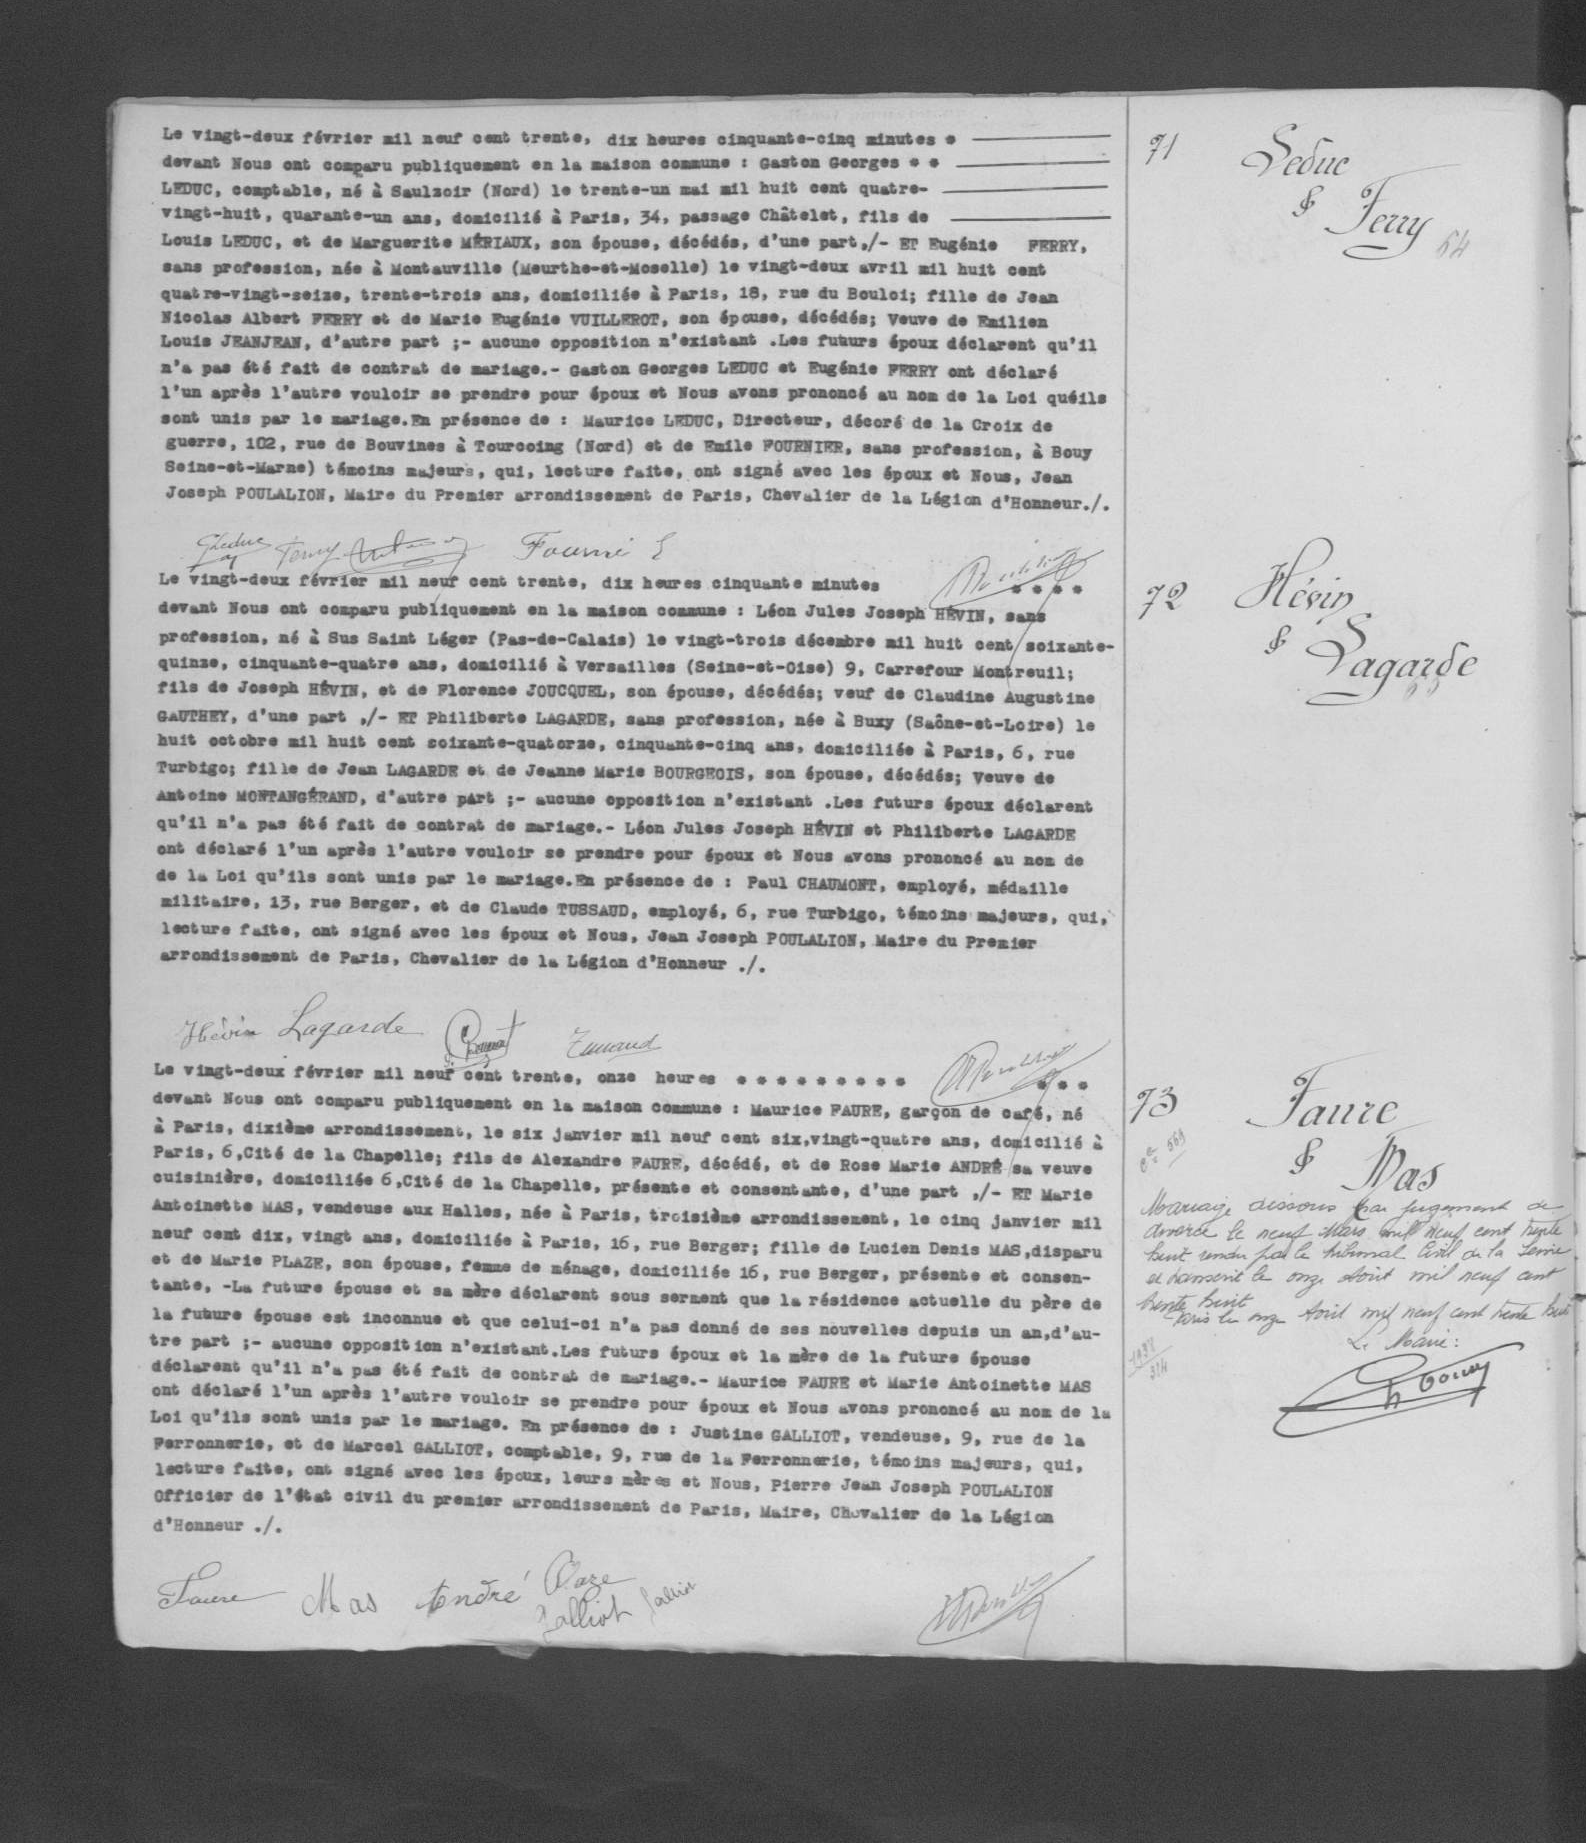Le vingt-deux février mil neuf cent trente, dix heures cinquante-cinq minutes * -
devant Nous ont comparu publiquement en la maison commune: Gaston Georges * * -
LEDUC, comptable, né à Saulzoir (Nord) le trente-un mai mil huit cent quatre-
vingt-huit, quarante-un ans, domicilié à Paris, 34, passage Châtelet, fils de -
Louis LEDUC, et de Marguerite MERIAUX, son épouse, décédés, d'une part ,-/ ET Eugénie FERRY,
sans profession, née à Montauville (Meurthe-et-Moselle) le vingt-deux avril mil huit cent
quatre-vingt-seize, trente-trois ans, domiciliée à Paris, 18, rue du Bouloi; fille de Jean
Nicolas Albert FERRY et de Marie Eugénie VUILLEROT, son épouse, décédés; veuve de Emilien
Louis JEANJEAN, d'autre part ;-aucune opposition n'existant. Les futurs époux déclarent qu'il
n'a pas été fait de contrat de mariage .-Gaston Georges LEDUC et Eugénie FERRY ont déclaré
l'un après l'autre vouloir se prendre pour époux et Nous avons prononcé au nom de la Loi quéils
sont unis par le mariage. En présence de: Maurice LEDUC, Directeur, décoré de la Croix de
guerre, 102, rue de Bouvines à Tourcoing (Nord) et de Emile FOURNIER, sans profession, à Bouy
Seine-et-Marne) témoins majeurs, qui, lecture faite, ont signé avec les époux et Nous, Jean
Joseph POULALION, Maire du Premier arrondissement de Paris, Chevalier de la Légion d'Honneur ./.
Le vingt-deux février mil neuf cent trente, dix heures cinquante minutes ****
devant Nous ont comparu publiquement en la maison commune: Léon Jules Joseph HEVIN, sans
profession, né à Sus Saint Léger (Pas-de-Calais) le vingt-trois décembre mil huit cent soixante-
quinze, cinquante-quatre ans, domicilié à Versailles (Seine-et-oise) 9, Carrefour Montreuil;
fils de Joseph HEVIN, et de Florence JOUCQUEL, son épouse, décédés; veuf de Claudine Augustine
GAUTHEY, d'une part ,/-ET Philiberte LAGARDE, sans profession, née à Buxy (Saône-et-Loire) le
huit octobre mil huit cent soixante-quatorze, cinquante-cinq ans, domiciliée à Paris, 6, rue
Turbigo; fille de Jean LAGARDE et de Jeanne Marie BOURGEOIS, son épouse, décédés; veuve de
Antoine MONTANGERAND, d'autre part ;-aucune opposition n'existant. Les futurs époux déclarent
qu'il n'a pas été fait de contrat de mariage .-Léon Jules Joseph HEVIN et Philiberte LAGARDE
ont déclaré l'un après l'autre vouloir se prendre pour époux et Nous avons prononcé au nom de
de la Loi qu'ils sont unis par le mariage. En présence de: Paul CHAUMONT, employé, médaille
militaire, 13, rue Berger, et de Claude TUSSAUD, employé, 6, rue Turbigo, témoins majeurs, qui,
lecture faite, ont signé avec les époux et Nous, Jean Joseph POULALION, Maire du Premier
arrondissement de Paris, Chevalier de la Légion d'Honneur ./.
Le vingt-deux février mil neuf cent trente, onze heures ****
devant Nous ont comparu publiquement en la maison commune: Maurice FAURE, garçon de café, né
à Paris, dixième arrondissement, le dix janvier mil neuf cent six, vingt-quatre ans, domicilié à
Paris, 6, Cité de la Chapelle; fils de Alexandre FAURE, décédé, et de Rose Marie ANDRE sa veuve
cuisinière, domiciliée 6, Cité de la Chapelle, présente et consentante, d'une part ,/-ET marie
Antoinette MAS, vendeuse aux Halles, née à Paris, troisième arrondisement, le cinq janvier mil
neuf cent dix, vingt ans, domiciliée à Paris, 16, rue Berger; fille de Lucien Denis MAS, disparu
et de Marie PLAZE, son épouse, femme de ménage, domiciliée 16, rue Berger, présente et consen-
tante,-La future épouse et sa mère déclarent sous serment que la résidence actuelle du père de
la future épouse est inconnue et que celui-ci n'a pas donné de ses nouvelles depuis un an, d'au-
tre part ;-aucune opposition n'existant. Les futurs époux et la mère de la future épouse
déclarent qu'il n'a pas été fait de contrat de mariage.-Maurice FAURE et Marie Antoinette MAS
ont déclaré l'un après l'autre vouloir se prendre pour époux et Nous avons prononcé au nom de la
Loi qu'ils sont unis par le mariage. En présence de: Justine GALLIOT, vendeuse, 9, rue de la
Ferronnerie, et de Marcel GALLIOT, comptable, 9, rue de la Ferronnerie, témoins majeurs, qui,
lecture faite, ont signé avec les époux, leurs mères et Nous, Pierre jean Joseph POULALION
Officier de l'état civil du premier arrondissement de Paris, Maire, Chevalier de la Légion
d'Honneur ./.
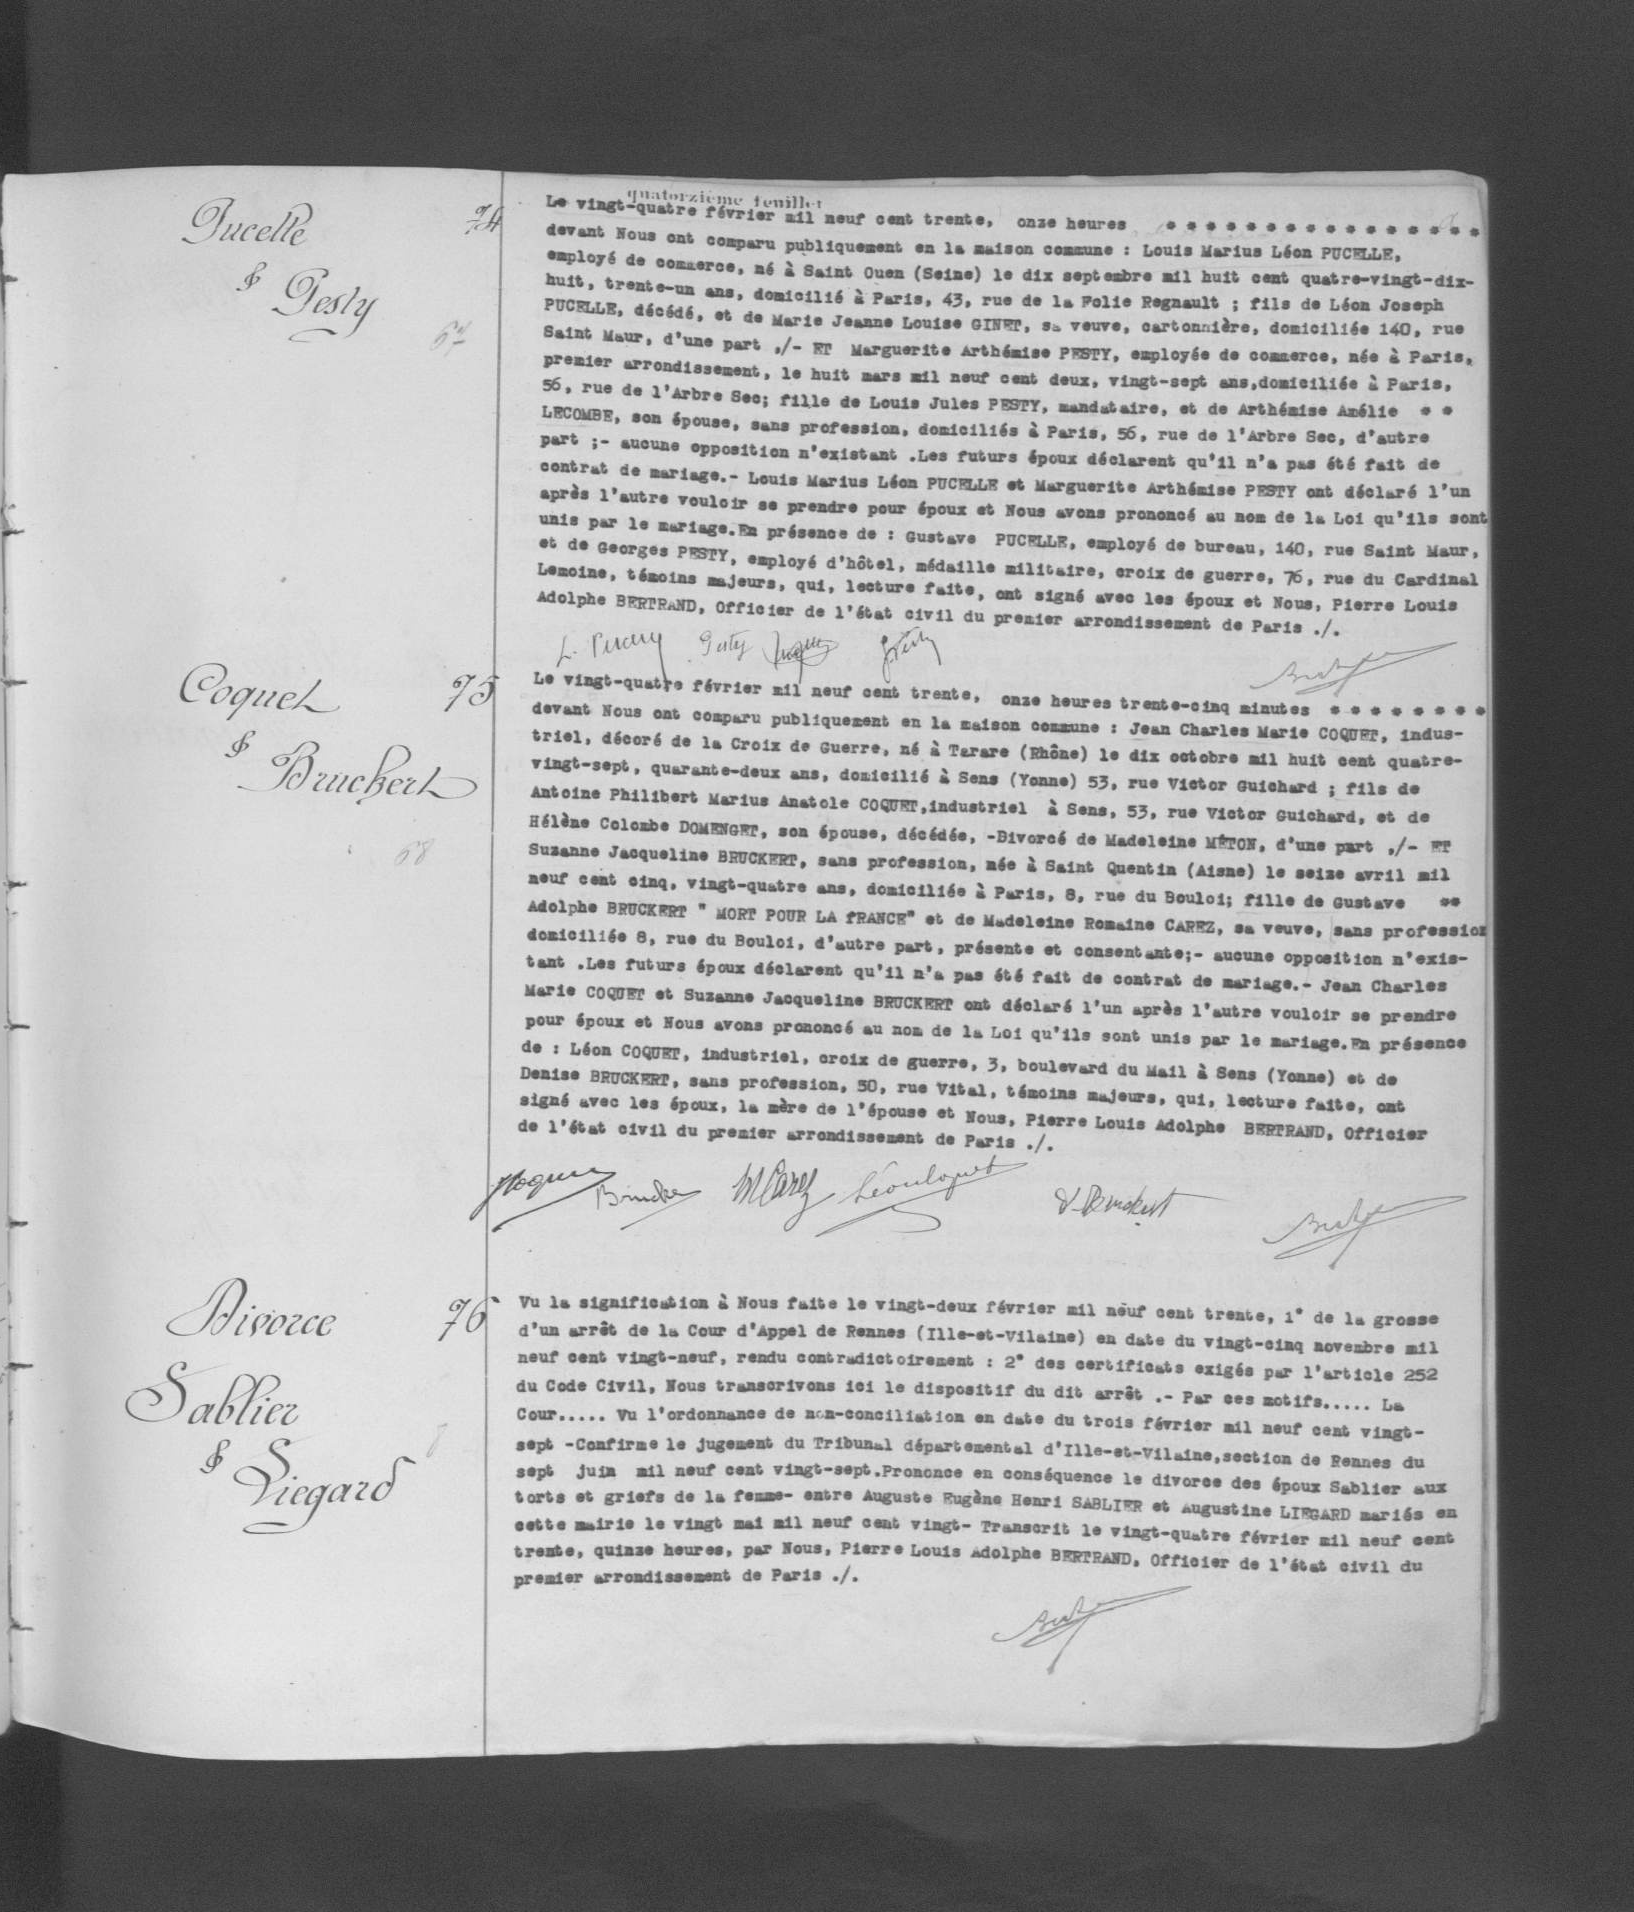Le vingt-quatre février mil neuf cent trente, onze heures ****
devant Nous ont comparu publiquement en la maison commune: Louis Marius Léon PUCELLE,
employé de commerce, né à Saint Ouen (Seine) le dix septembre mil huit cent quatre-vingt-dix-
huit, trente-un ans, domicilié à Paris, 43, rue de la Folie Regnault; fils de Léon Josep
PUCELLE, décédé, et de Marie Jeanne Louise GINET, sa veuve, cartonnière, domiciliée 140, rue
Saint Maur, d'une part ,/-ET Marguerite Arthémise PESTY, employée de commerce, née à Paris,
premier arrondissement, le huit mars mil neuf cent deux, vingt-sept ans, domiciliée à Paris,
56, rue de l'Arbre Sec; fille de Louis Jules PESTY, mandataire, et de l'Arthémise Amélie * *
LECOMBE, son épouse, sans profession, domiciliés à Paris, 56, rue de l'Arbre Sec, d'autre
part ;-aucune opposition n'existant. Les futurs époux déclarent qu'il n'a pas été fait de
contrat de mariage .-Louis Marius Léon PUCELLE et Marguerite Arthémise PESTY ont déclaré l'un
après l'autre vouloir se prendre pour époux et Nous avons prononcé au nom de la Loi qu'ils sont
unis par le mariage. En présence de: Gustave PUCELLE, empoyé de bureau, 140, rue Saint Maur,
et de Georges PESTY, employé d'hôtel, médaille militaire, croix de guerre, 76, rue du Cardinal
Lemoine, témoins majeurs, qui, lecture faite, ont signé avec les époux et Nous, Pierre Louis
Adolphe BERTRAND, Officier de l'état civil du premier arrondissement de Paris ./.
Le vingt-quatre février mil neuf cent trente, onze heures trente-cinq minutes ****
devant Nous ont comparu publiquement en la maison commune: Jean Charles Marie COQUET, indus-
triel, décoré de la Croix de Guerre, né à Tarare (Rhône) le dix octobre mil huit cent quatre-
vingt-sept, quarante-deux ans, domicilié à Sens (Yonne) 53, rue Victor Guichard; fils de
Antoine Philibert Marius Anatole COQUET, industriel à Sens, 53, rue Victor Guichard, et de
Hélène Colombe DOMENGET, son épouse, décédée,-Divorcé de Madeleine METON, d'une part ,/-ET
Suzanne Jacqueline BRUCKERT, sans profession, née à Saint Quentin (Aisne) le seize avril mil
neuf cent cinq, vingt-quatre ans, domiciliée à Paris, 8, rue du Bouloi; fille de Gustave **
Adolphe BRUCKERT 'MORT POUR LA FRANCE" et de Madeleine Romaine CAREZ, sa veuve, sans profession
domiciliée 8, rue du Bouloi, d'autre part, présente et consentante ;-aucune opposition n'exis-
tant. Les futurs époux déclarent qu'il n'a pas été fait de contrat de mariage .-Jean Charles
Marie COQUET et Suzanne Jacqueline BRUCKERT ont déclaré l'un après l'autre vouloir se prendre
pour époux et Nous avons prononcé au nom de la Loi qu'ils sont unis par le mariage. En présence
de: Léon COQUET, industriel, croix de guerre, 3, boulevard du Mail à Sens (Yonne) et de
Denise BRUCKERT, sans profession, 50, rue Vital, témoins majeurs, qui, lecture faite, ont
signé avec les époux, la mère de l'épouse et Nous, Pierre Louis Adolphe BERTRAND, Officier
de l'état civil du premier arrondissement de Paris ./.
Vu la signification à Nous faite le vingt-deux février mil neuf cent trente, 1° de la grosse
d'un arrêt de la Cour d'Appel de Rennes (Ille-et-Vilaine) en date du vingt-cinq novembre mil
neuf cent vingt neuf, rendu contradictoirement: 2° des certificats exigés par l'article 252
du Code Civil Nous transcrivons ici le dispositif du dit arrêt .-Par ces motifs ..... La
Cour ..... Vu l'ordonnance de non-conciliation en date du trois février mil neuf cent vingt-
sept-Confirme le jugement du Tribunal départemental d'Ille-et-Vilaine, section de Rennes du
sept juin mil neuf cent vingt-sept. Prononce en conséquence le divorce des époux Sablier aux
torts et griefs de la femme-entre Auguste Eugène Henri SABLIER et Augustine LIEGARD mariés en
cette maire le vingt mai mil neuf cent vingt-Transcrit le vingt-quatre février mil neuf cent
trente, quinze heures, par Nous, Pierre Louis Adolphe BERTRAND, Officier de l'état civil du
premier arrondissement de Paris ./.